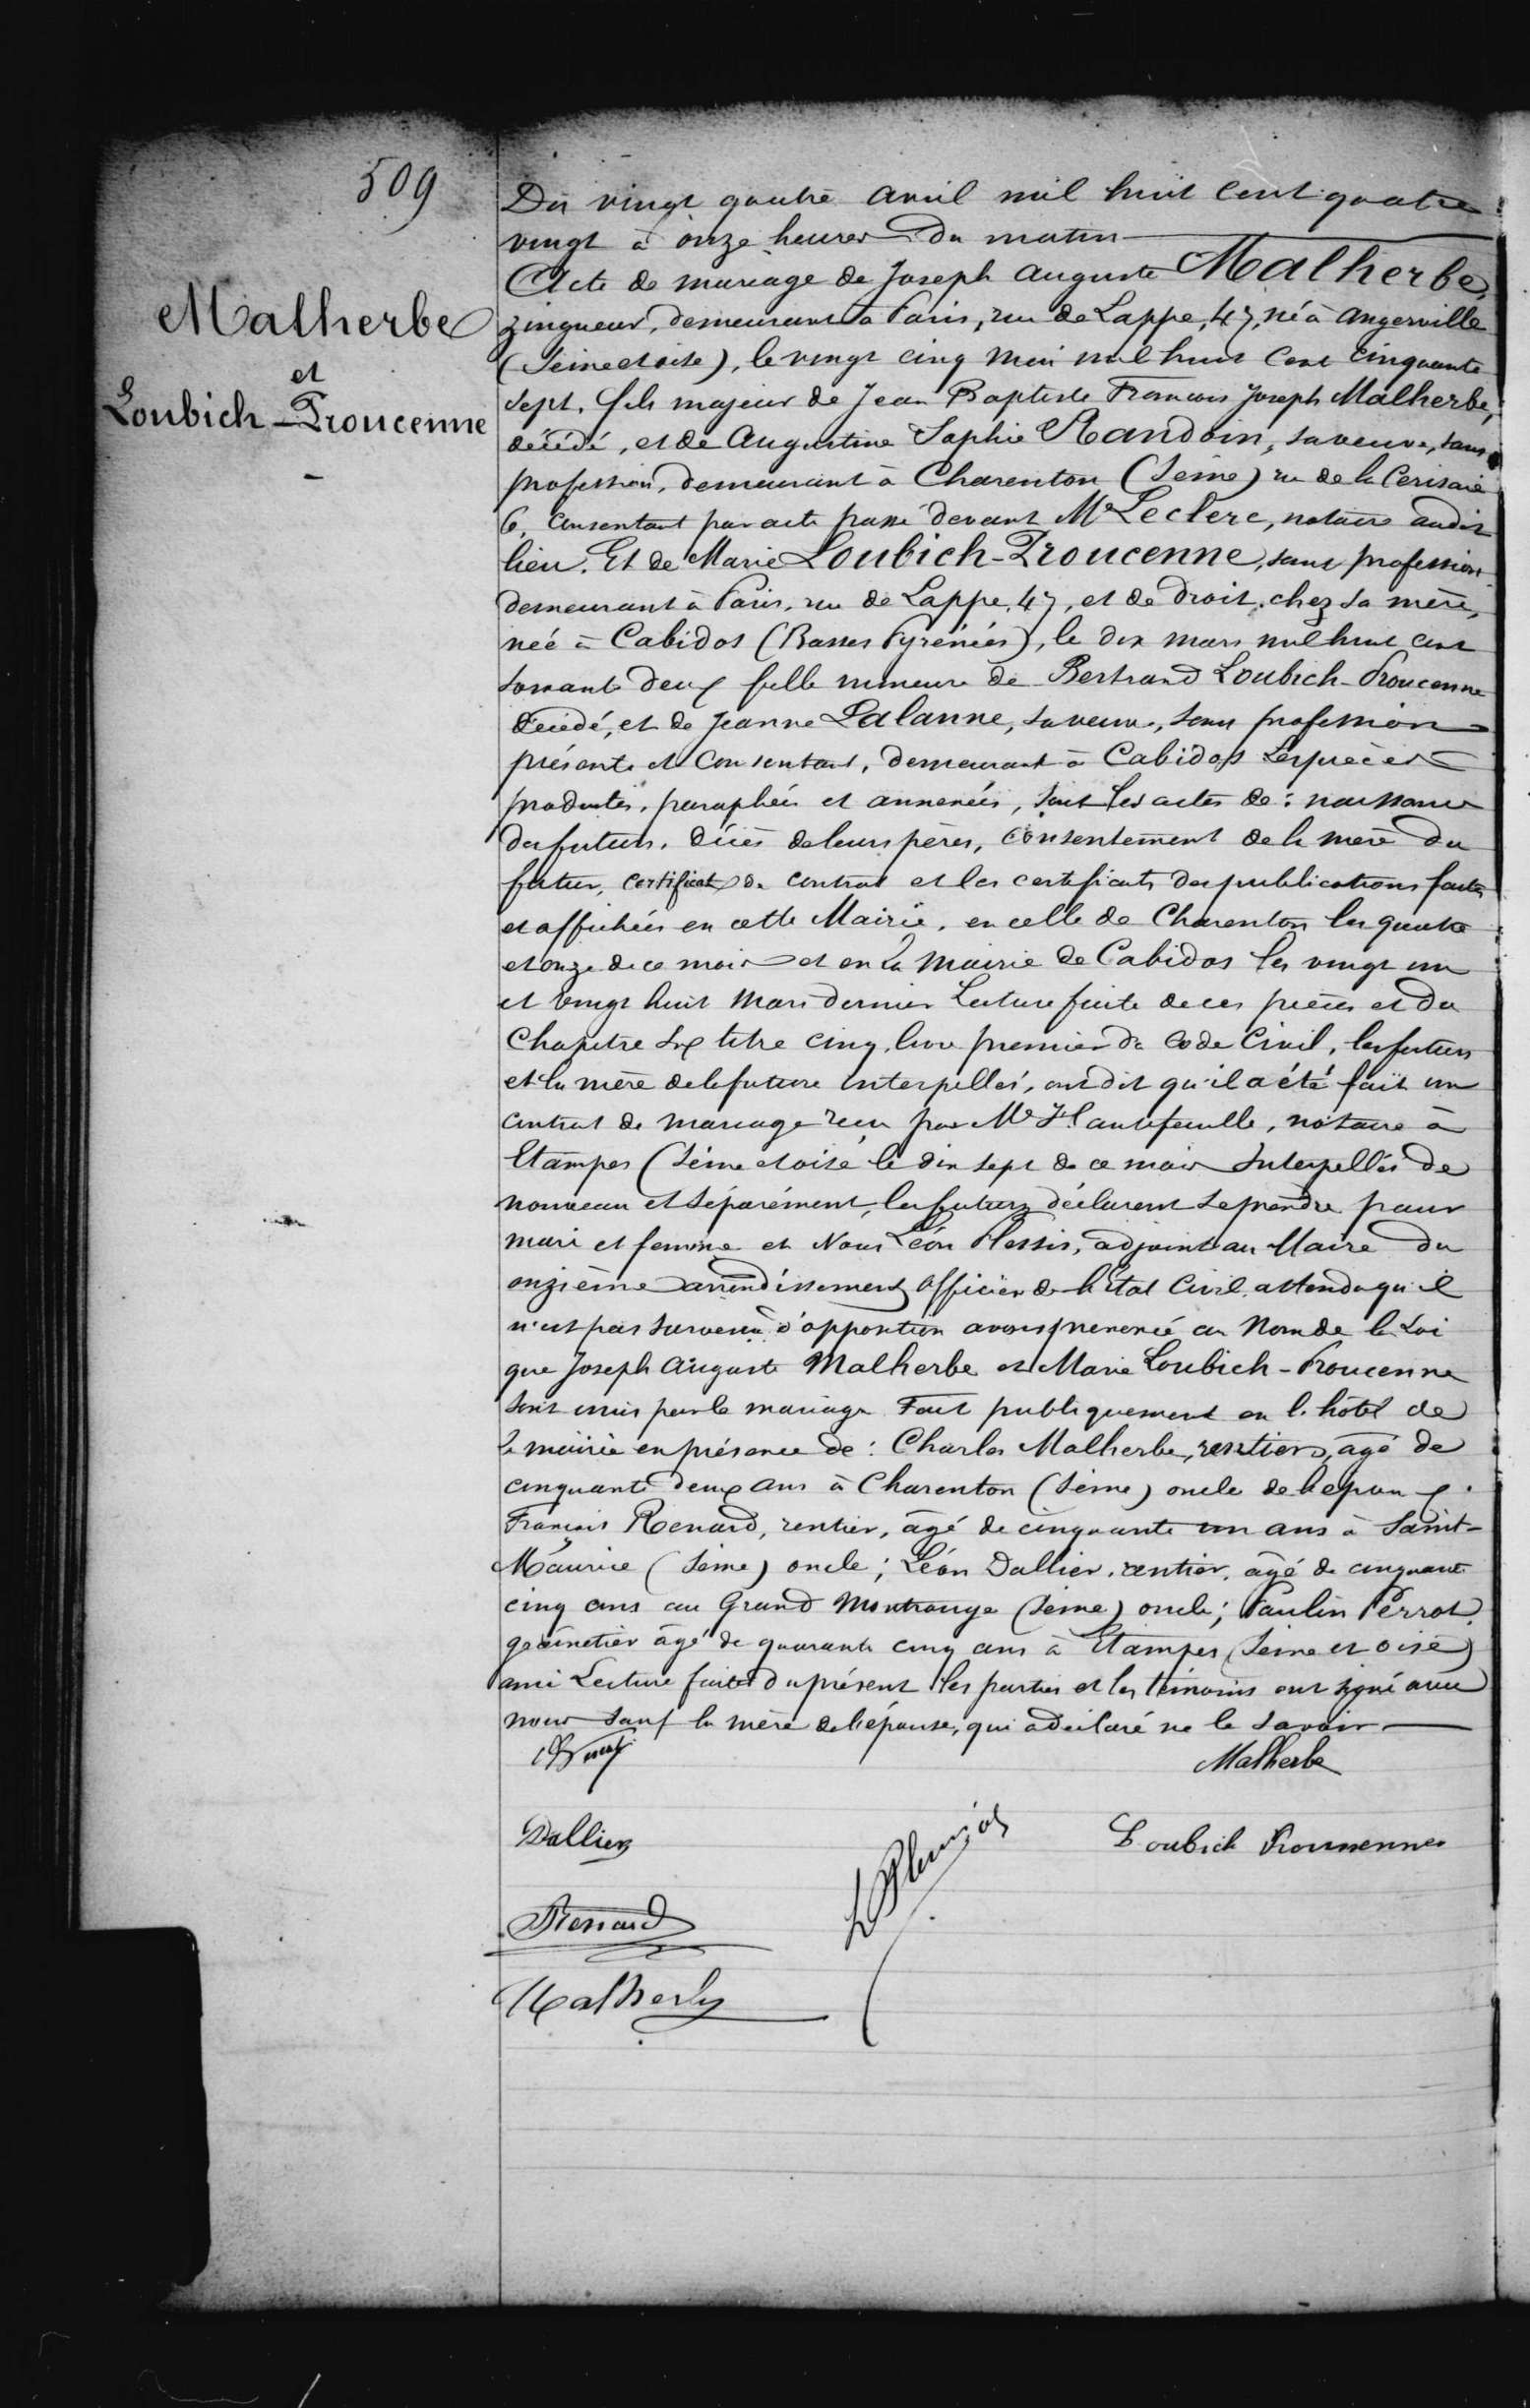309
Malherbe
et
Loubich Proucenne
Du vingt quatre avril mil huit cent quatre
vingt à onze heures du matin
Acte de mariage de Joseph Auguste Malherbes
zinggueur, demeurant à Paris, rue de Lappe, 43, né à Angerville
(Seine et Oise), le vingt cinq mai mil huit cent cinquante
sept, fils majeur de Jean Baptiste Françoise Joseph Malherbe,
décédé, et de Augustine Sophie Aandoin, sa veuve, sans
profession, demeurant à Charenton (Seine) rue de le Cerisaie
6, consentant par acte passé devant M°Leclerc notaire audit
lieu. Et de Marie Loubich-Proucenne, sans profession
demeurant à Paris, rue de Lappe, 47, et de droit, chez sa mère,
née à Cabidos (Basses Pyrénées), le dix mars mil huit cent
soixante deux fille ineure de Bertrand Loubich Proucenne
décédé, et de Jeanne Lalanne, sa veuve, sans profession
présents et consentants, demeurant à Cabidos Les pièces
produites, paraphées et annexées, dont les actes de: naissance
des futurs, décès de leurs pères, consentement de la mère du
futur, certificat de contrat et les certificat des publications faites
et affichées en cette Mairie, en celle de Charenton les quatre
et onze mai et en la mairie de Cabidos les vingt un
et vingt huit Mars dernier. Lecture faite de ces pièces et du
chapitre six titre cinq, livre premier du code civil, les futurs
et la mère de la future interpellés, ont dit qu'il a été fait un
contrat de mariage reçu par Me Hautefeuille, notaire à
Etampes (Seine et Oise le dix sept de ce mois Interpellés de
nouveau et séparément, les futurs déclarent se prendre pour
mari et femme et Nous Léon Hessis, adjoint au Maire du
onzième arrondissement officier de l'état civil attendu qu'il
n'ont pas survenu d'opposition vous prononcé au nom de la Loi
que Joseph Auguste Malherbe et Marie Loubich-Troucenne
sont unis par le mariage. Fait publiquement en l'hôtel de
la mairie en présence de: Charles Malherbe, rentier, âgé de
cinquante deux ans à Charenton (Seine) oncle de l'époux &
François Renard, rentier, âgé de cinquante un ans à Saint-
Maurice (Seine) oncle; Léon Dallier, rentier, âgé de cinquante
cinq ans au Grand Montrouge (Seine) oncle; Paulin Perrot
grainetier âgé de quarante cinq ans à Etampes (Seine et Oise)
ami Lecture faite du présent les parties et les témoins ont signé avec
nous sauf la mère de l'épouse qui a déclaré ne le savoir
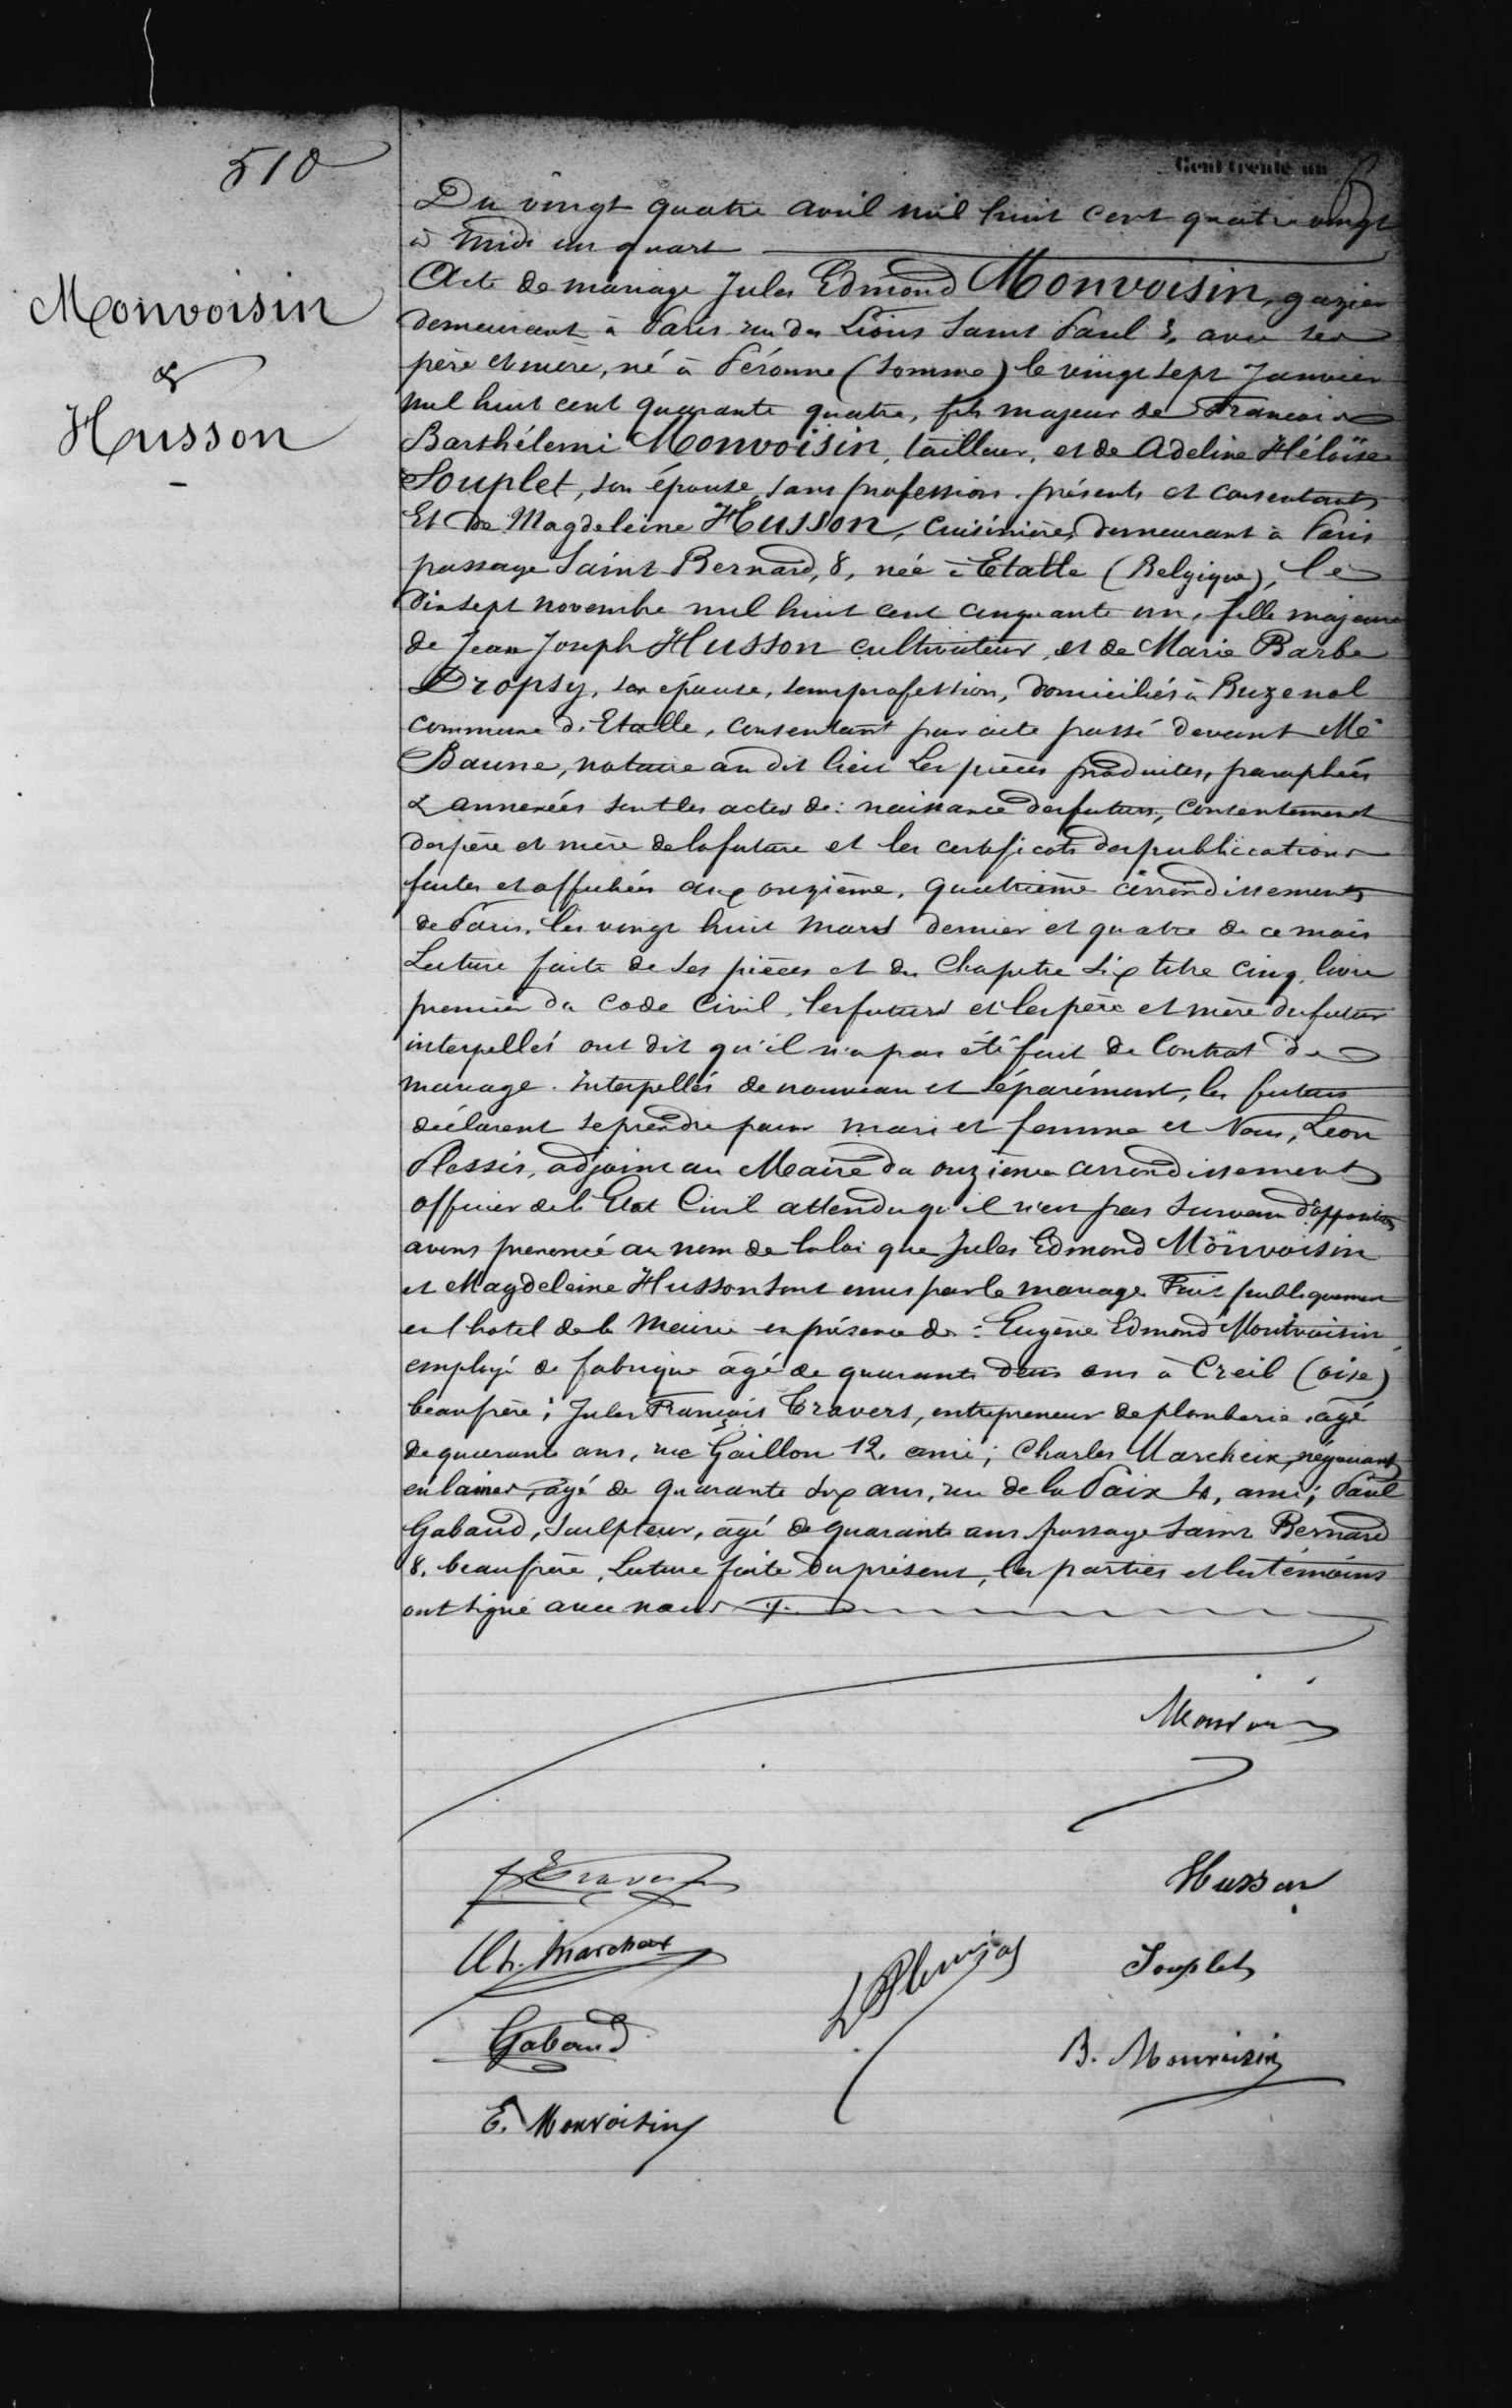510
Monvoisin
&
Husson
Du vingt quatre avril mil huit cent quatre vingt
à midi un quart -
Acte de mariage Jules Edmond Monvoisin, gazier
demeurant à Paris rue des Lions Saint Paul 3, avec ses
père et mère, né à Péronne (Somme) le vingt sept Janvier
mil huit cent quarante quatre, fils majeur de François
Barthélemi Monvoisin, tailleur, et de Adeline Héloïse
Souplet, son épouse, sans profession, présents et consentants
Et de Madgeleine Husson, cuisinière demeurant à Paris
passage Saint Bernard, 8, née à Etable (Belgique), le
dix sept novembre mil huit cent cinquante un, fille majeure
de Jean Joseph Husson cultivateur et de Marie Barbe
Dropsy son épouse, sans profession, domicilié à Ruzenal
commune d'Etable, consentant par acte passé devant Me
Baune, notaire au dit lieu Les pièces produites, paraphées
& annexées sont les actes de: naissance des futurs, consentement
des père et mère de la future et les certificats de publications
faites et affichées aux onzième, quatrième arrondissements
de Paris, les vingt huit mars dernier et quatre de ce mois
Lecture faite de ses pièces et du chapitre six titre cinq, livre
premier du code civil, les futurs et les père et mère du futur
interpellés ont dit qu'il n'a pas été fait de contrat de
mariage, interpellés de nouveau et séparément, les futurs
déclarent se prendre pour mai et femme et Nous, Léon
Plessis, adjoint au Maire du onzième arrondissement
officier de l'Etat Civil attendu qu'il n'est pas survenu d'opposition
avons prononcé au nom de la loi que Jules Edmond Monvoisin
et Magdeleine Husson sont unis par le mariage. Fait publiquement
en l'hotel de la mairie en présence de: Eugène Edmond Montvoisin
employé de fabrique âgé de quarante deux ans à Creil (oise)
beaupère; Jules François Tavers, entrepreneur de plomberie agé
de quarante ans, rue Gaillon 12 ami; Charles Marcheix, négociant
en laine, agé de quarante six ans, rue de la Paix 4, ami; Paul
Gabaud, sculpteur, âgé de quarante ans passage Saint Bernard
8. beau-frère, Lecture faite du présent, les parties et les témoins
ont signé avec nous ./.